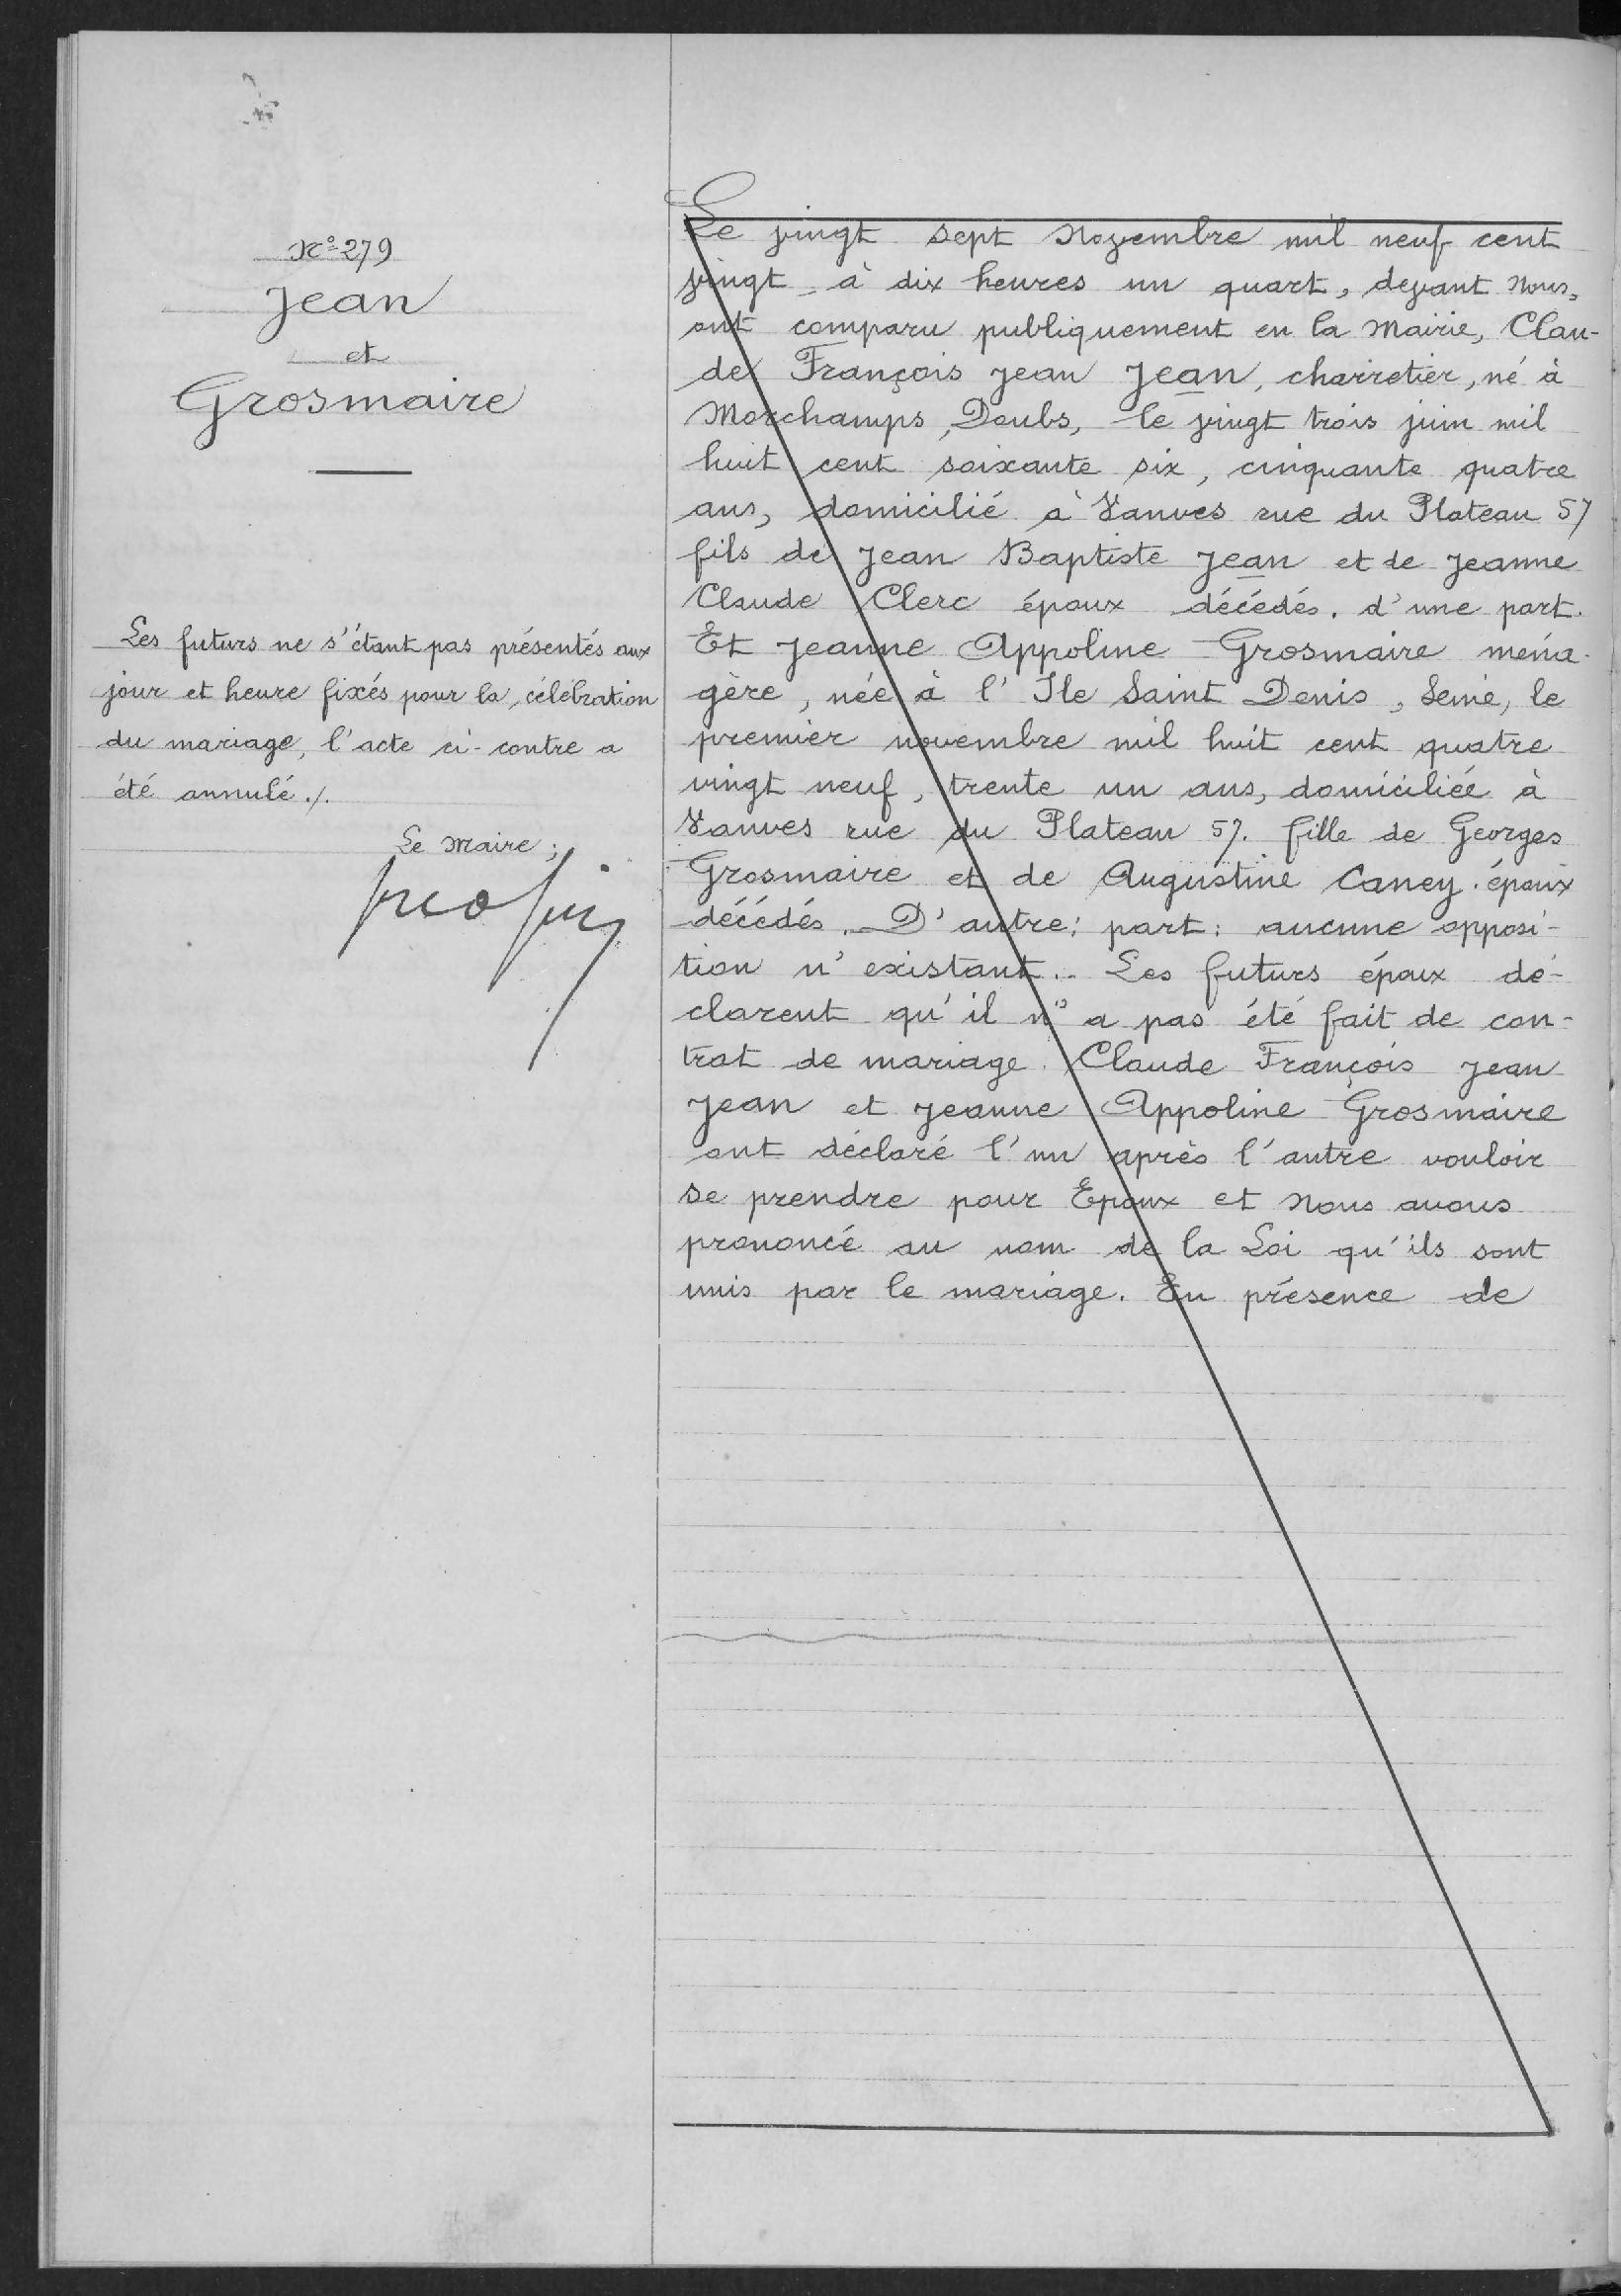n°279
Jean
et
Grosmaire
Le vingt sept novembre mil neuf cent
vingt à dix heures un quart, devant nous
ont comparu publiquement en la Mairie, Clau-
de François jean jean, charretier, né à
Marchamps, Doubs, le vingt trois juin mil
huit cent soixante six, cinquante quatre
ans, domicilié à Vanves rue du Plateau 57
fils de Jean Baptiste Jean et de Jeanne
Claude Clerc époux décédés. d'une part.
Et Jeanne Appoline Grosmaire ména-
gère, née à l'Ile Saint Denis, Seine, le
premier novembre mil huit cent quatre
vingt neuf, trente un ans, domiciliée à
Vanves rue du Plateau 57. fille de Georges
Grosmaire et de Augustine Caney. épuox
décédés. D'autre: part: aucune opposi-
tion n'existant. Les futurs époux dé-
clarent qu'il n'a pas été fait de con-
trat de mariage. Claude François Jean
Jean et Jeanne Appoline Grosmaire
ont déclaré l'un après l'autre vouloir
se prendre pour Epoux et nous avons
prononcé au nom de la Loi qu'ils sont
unis par le mariage. En présence de
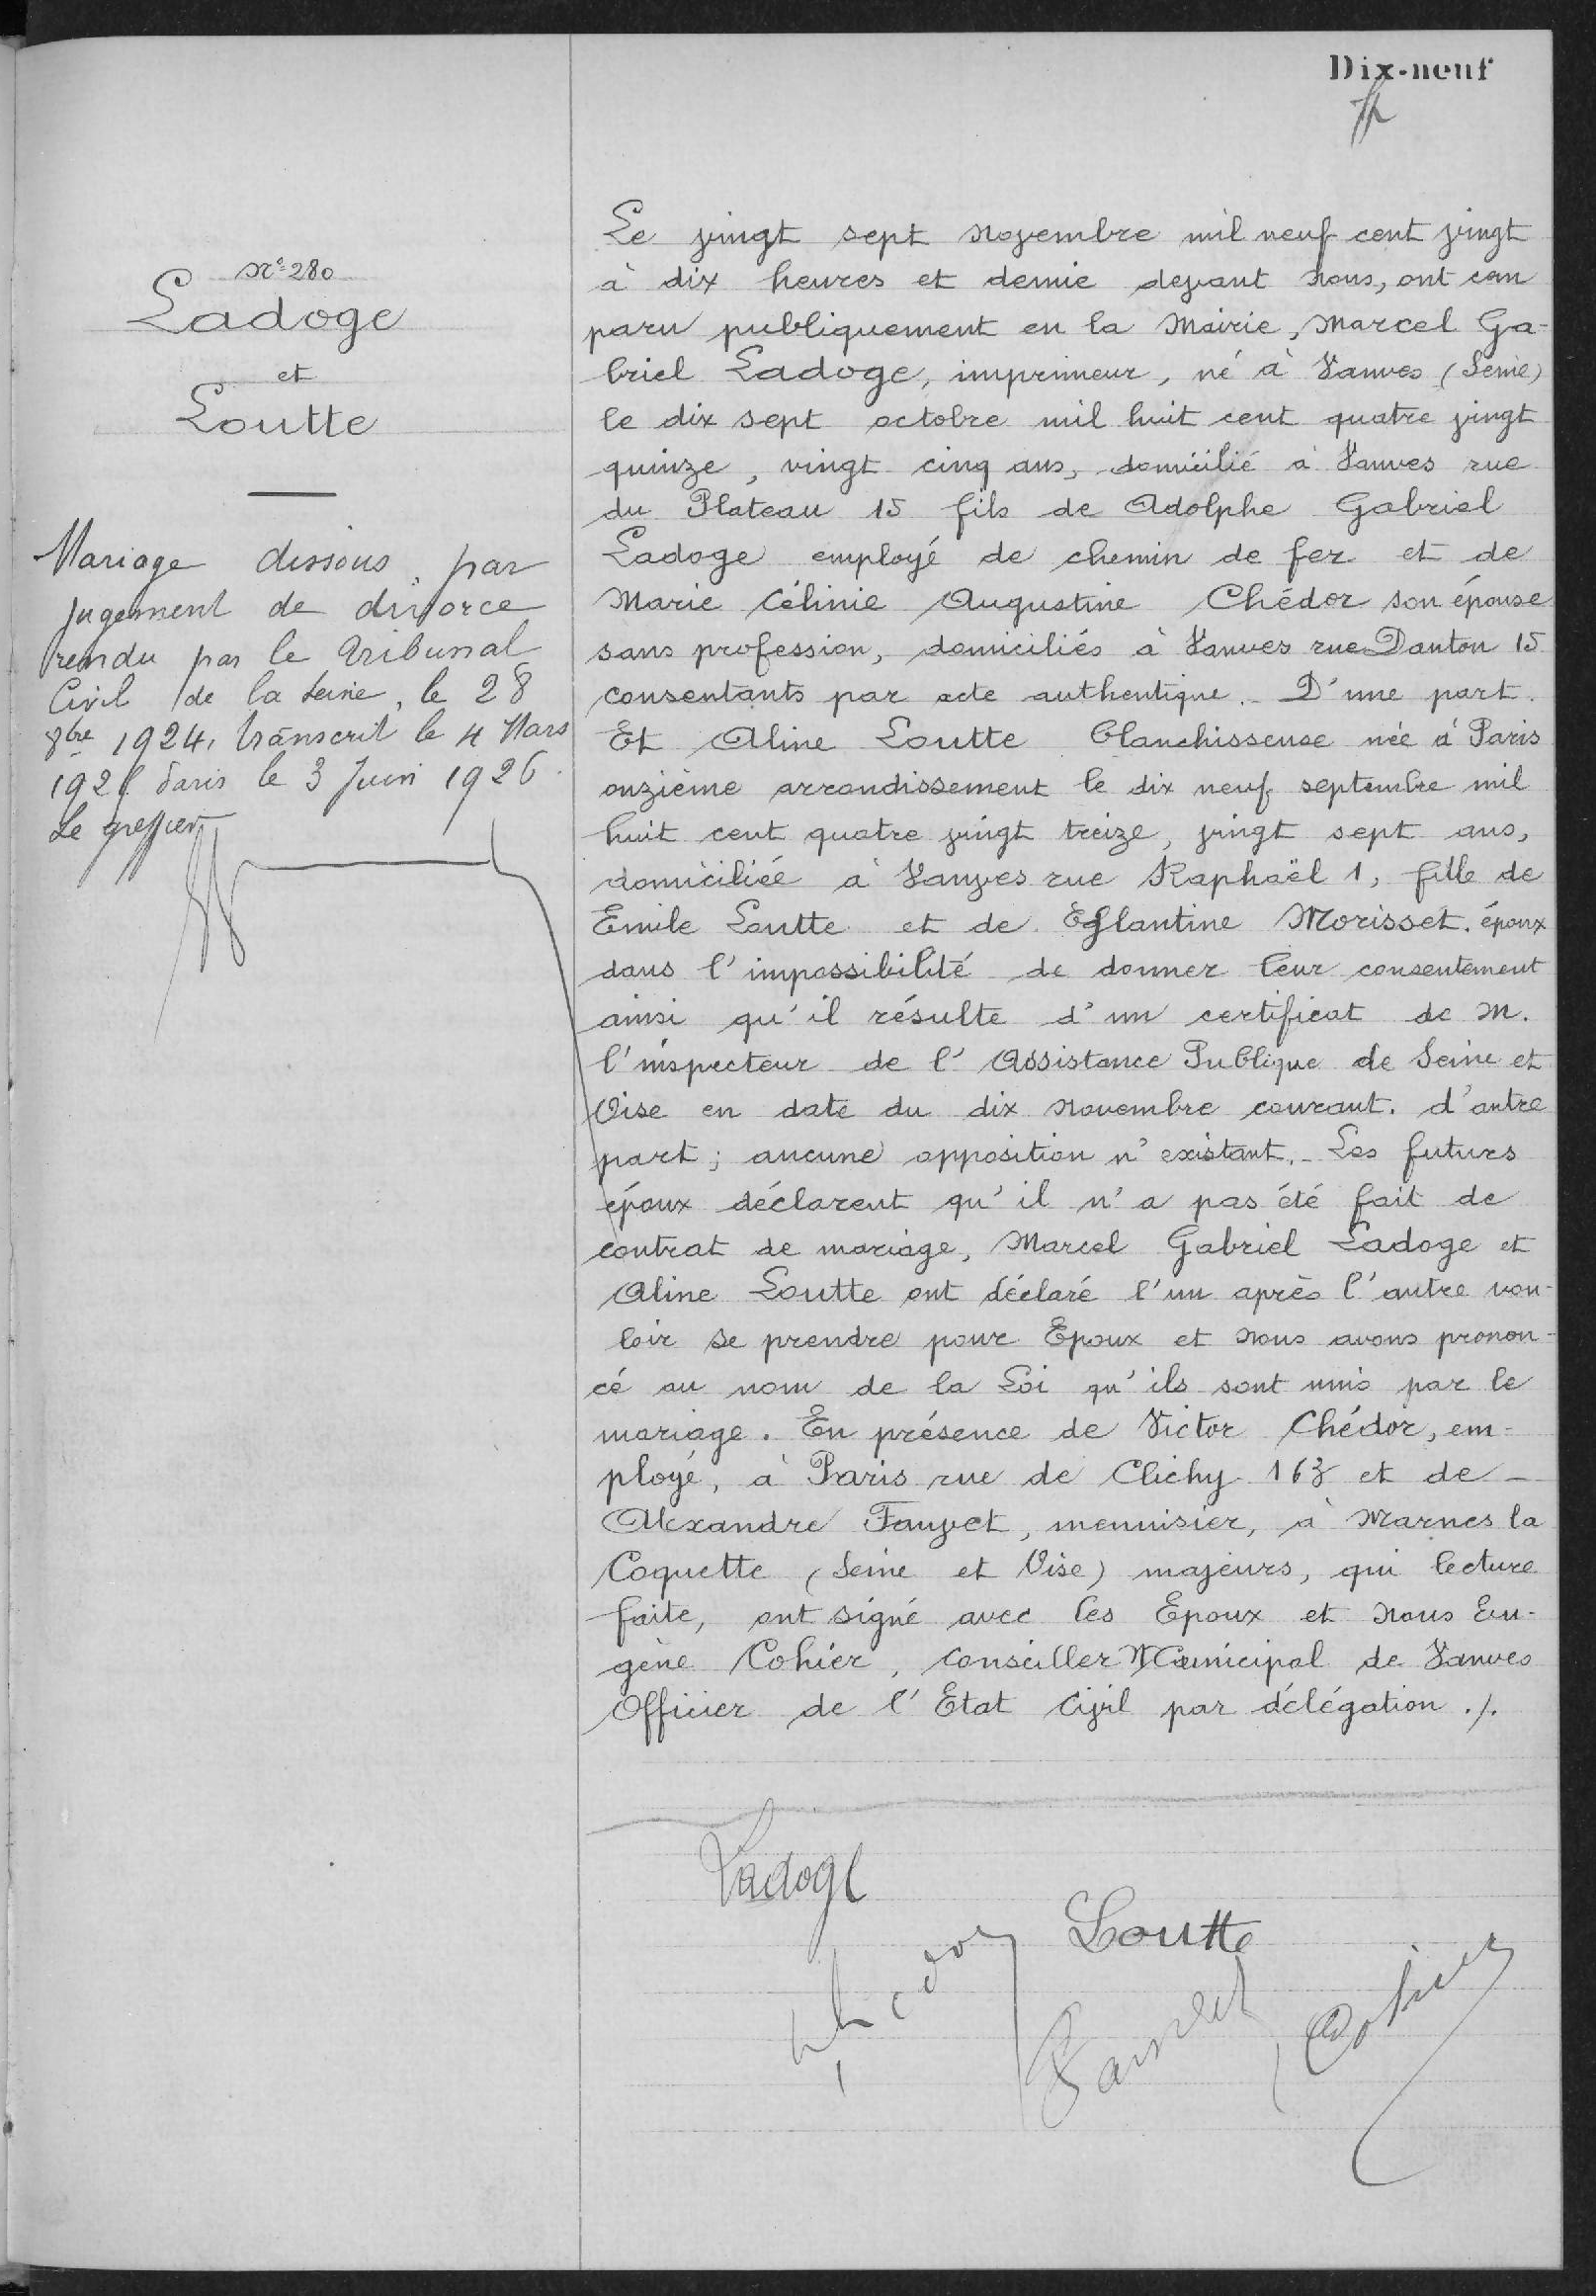n°280
Ladoge
et
Loutte
Le vingt sept novembre mil neuf cent vingt
à dix heures et demie devant nous, ont com
paru publiquement en la Mairie, Marcel Ga-
breil Ladoge, impimeur, né à Vanves (seine)
le dix sept octobre mil huit cent quatre vingt
quinze, vingt cinq ans, domicilié à Vanves rue
du Plateau 15 fils de Adolphe Gabriel
Ladoge employé de chemin de fer et de
Marie Célinie Augustine, Chédor son épouse
sans profession, domiciliés à Vanves rue Danton 15
consentants par acte authentique. D'une part.
Et Aline Loutte blanchisseuse née à Paris
onzième arrondissement le dix neuf septembre mil
huit cent quatre vingt treize, vingt sept ans,
domiciliée à Vanves rue Raphaël 1, fille de
Emile Lautte et de Eglantine Morisset. époux
dans l'impossibilité de donner leur consentement
ainsi qu'il résulte d'un certifcat de m.
l'inspecteur de l'Assistance Publique de Seine et
Oise en date du dix novembre courant. d'autre
part; aucune opposition n'existant. Les futurs
époux déclarent qu'il n'a pas été fait de
contrat de mariage, Marcel Gabriel Ladoge et
Aline Loutte ont déclaré l'un après l'autre vou-
loir se prendre pour Epoux et nous avons pronon-
cé au nom de la Loi qu'ils sont unis par le
mariage. En présence de Victor, Chédor, em-
ployé, à Paris rue de Clichy. 163 et de-
Alexandre Fauyet, menuisier, à Marnes la
Coquette (Seine et Oise) majeurs, qui lecture
faite, ont signé avec les Epoux et Nous Eu-
gène Cohier, conseille Municipal de Vanves
Officier de l'Etat Civil par délégation./.